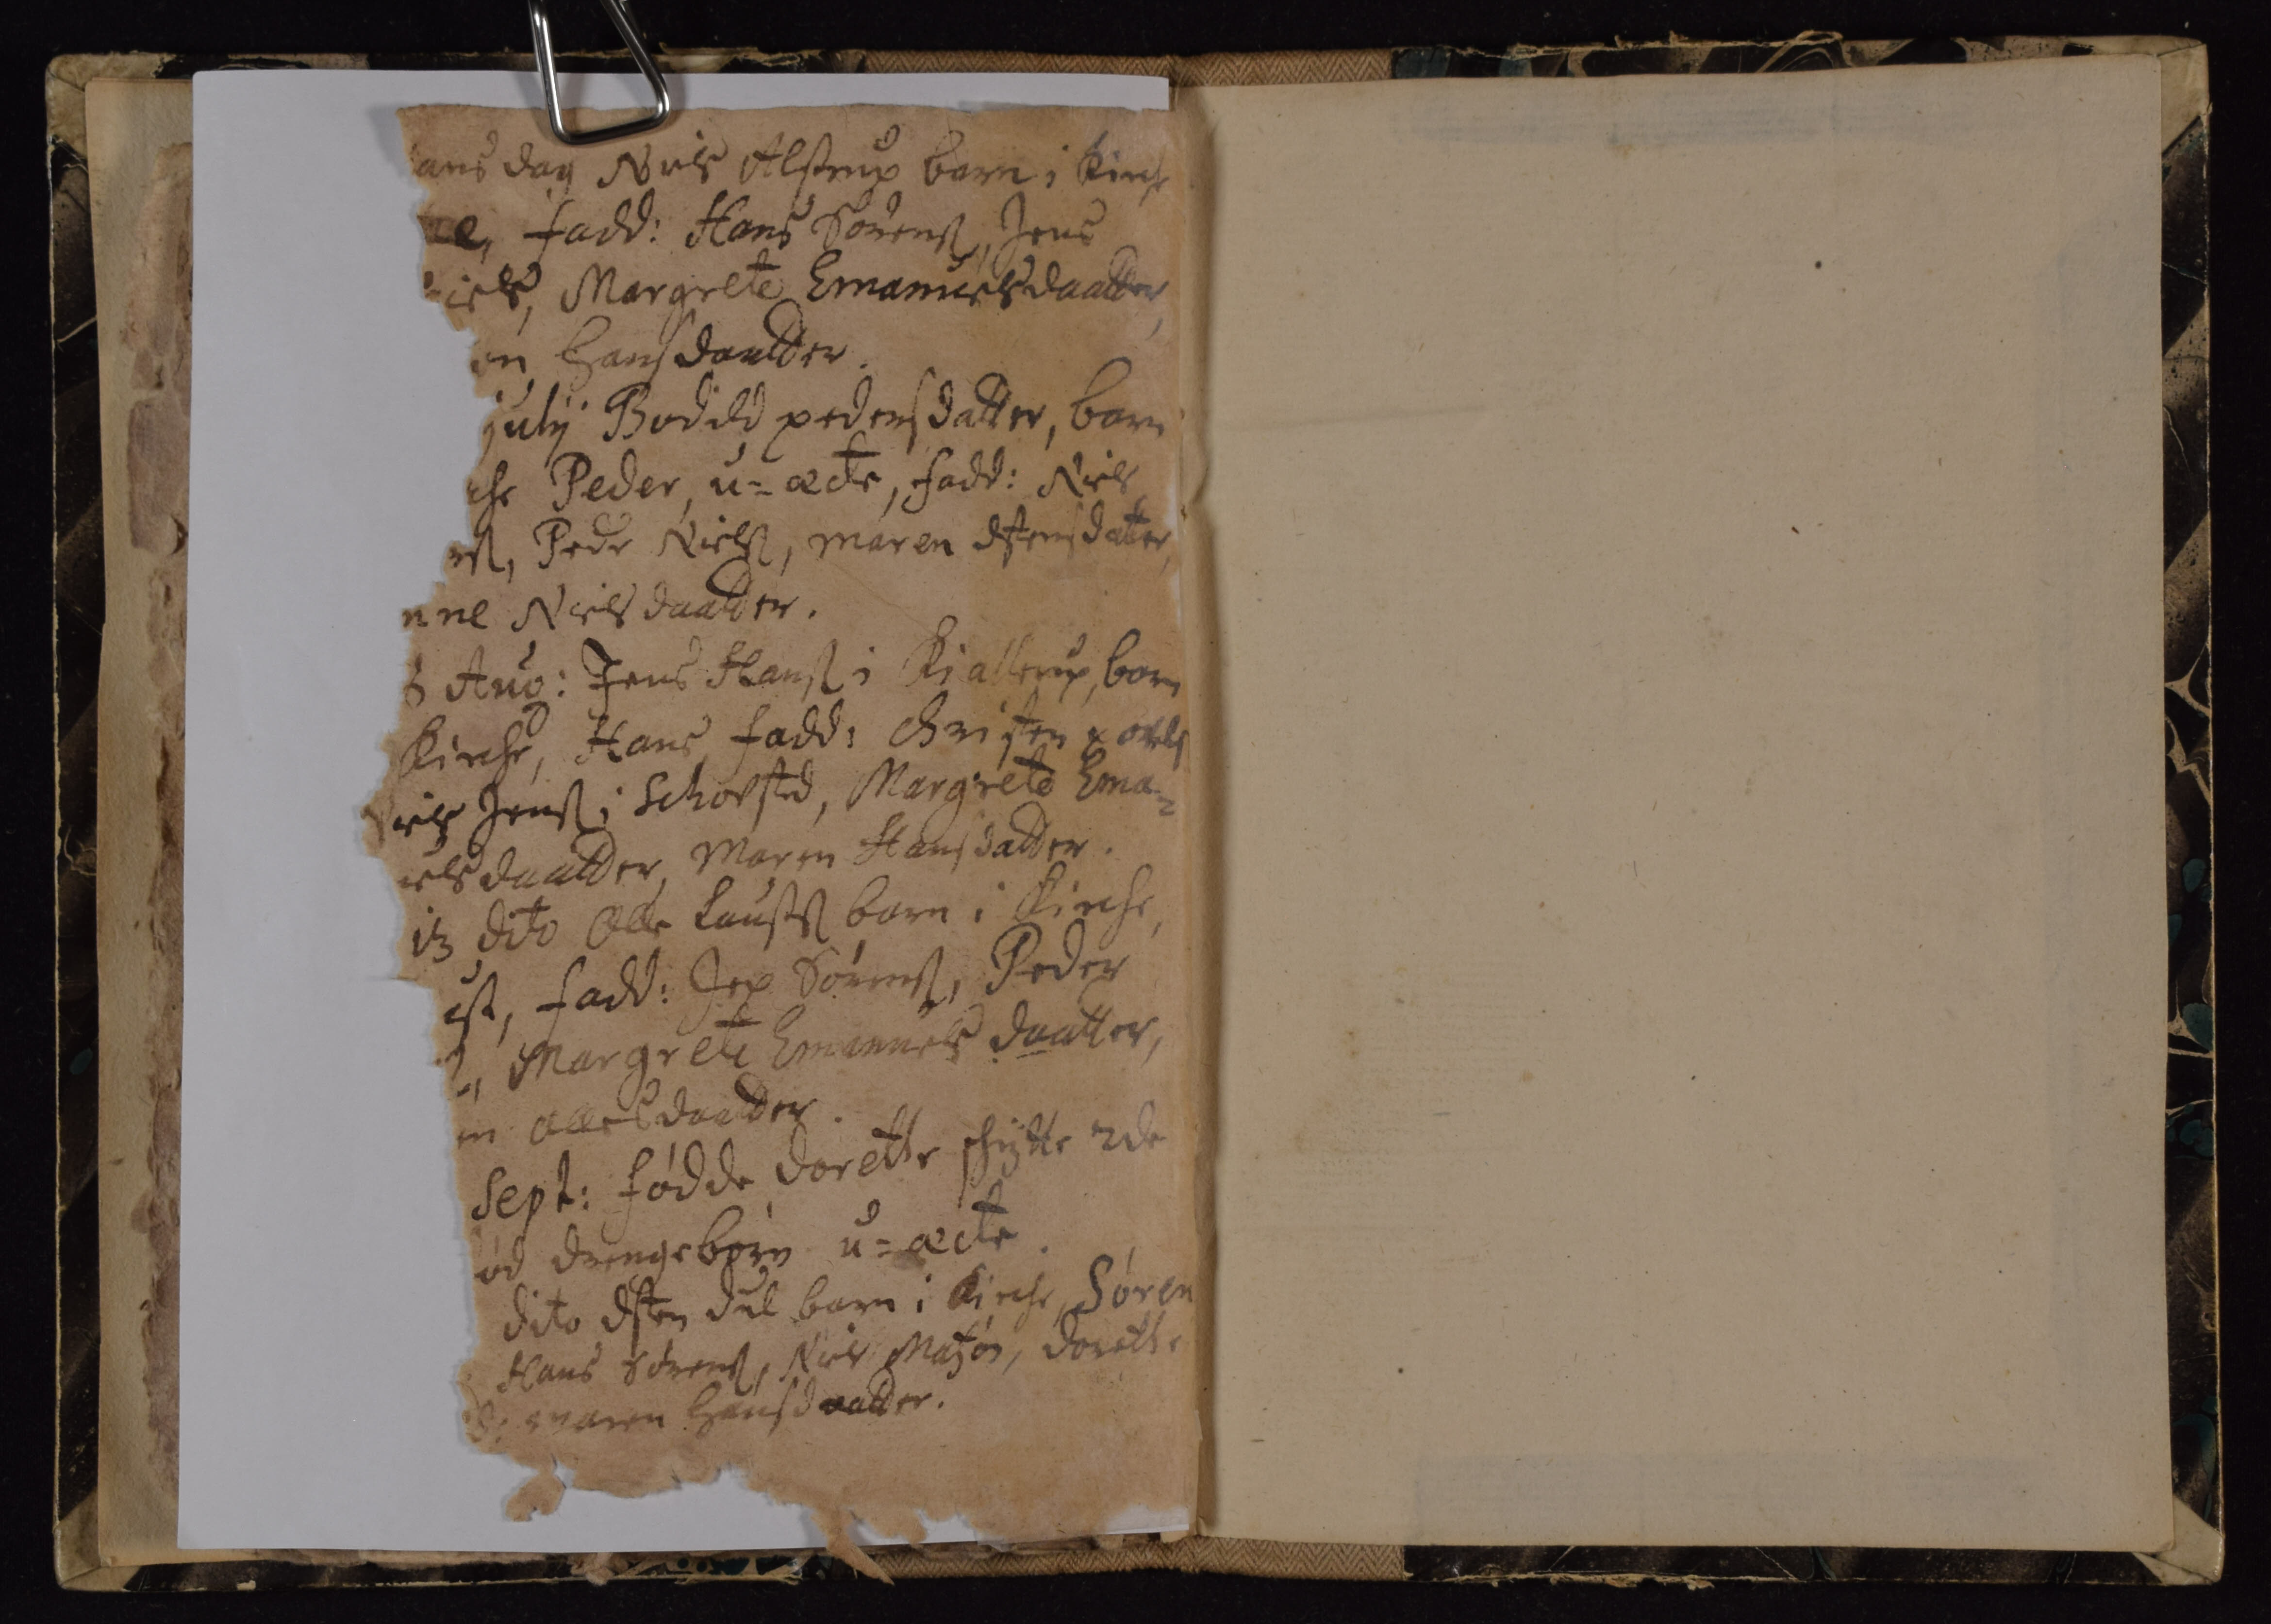ansdag Niels Alstrup barn i Kirche
e Fadd. Hans Sørens, Jens
iels Margrete Emanuelsdaatter
n Hansdaatter.
Julij Bodild pedersdatter, barn
che Peder u=æcte, Fadd; Niels
##s Pedr Niels, Maren ##stensdatter
nne Nielsdaatter.
## Aug: Jens Hans i Kiallerup barn
Kirche, Hans Fadd: Christen ##
iels Jens i Schovsted, Margrete Ema¬
elsdaatter Maren Hansdatter.
## dito Olle Lausts barn i Kirche
##st, Fadd: Jep Sørens; Peder
Margrete Emanuelsdaatter,
n Ollesdaatter.
Sept: Fødde Dorette shytte 2de
ød drengebærn u-æcte
dito ##sten Jul barn i Kirche Søren
Hans Sørens, Niels Matzøn, Dorethe
## Maren Hansdaatter.